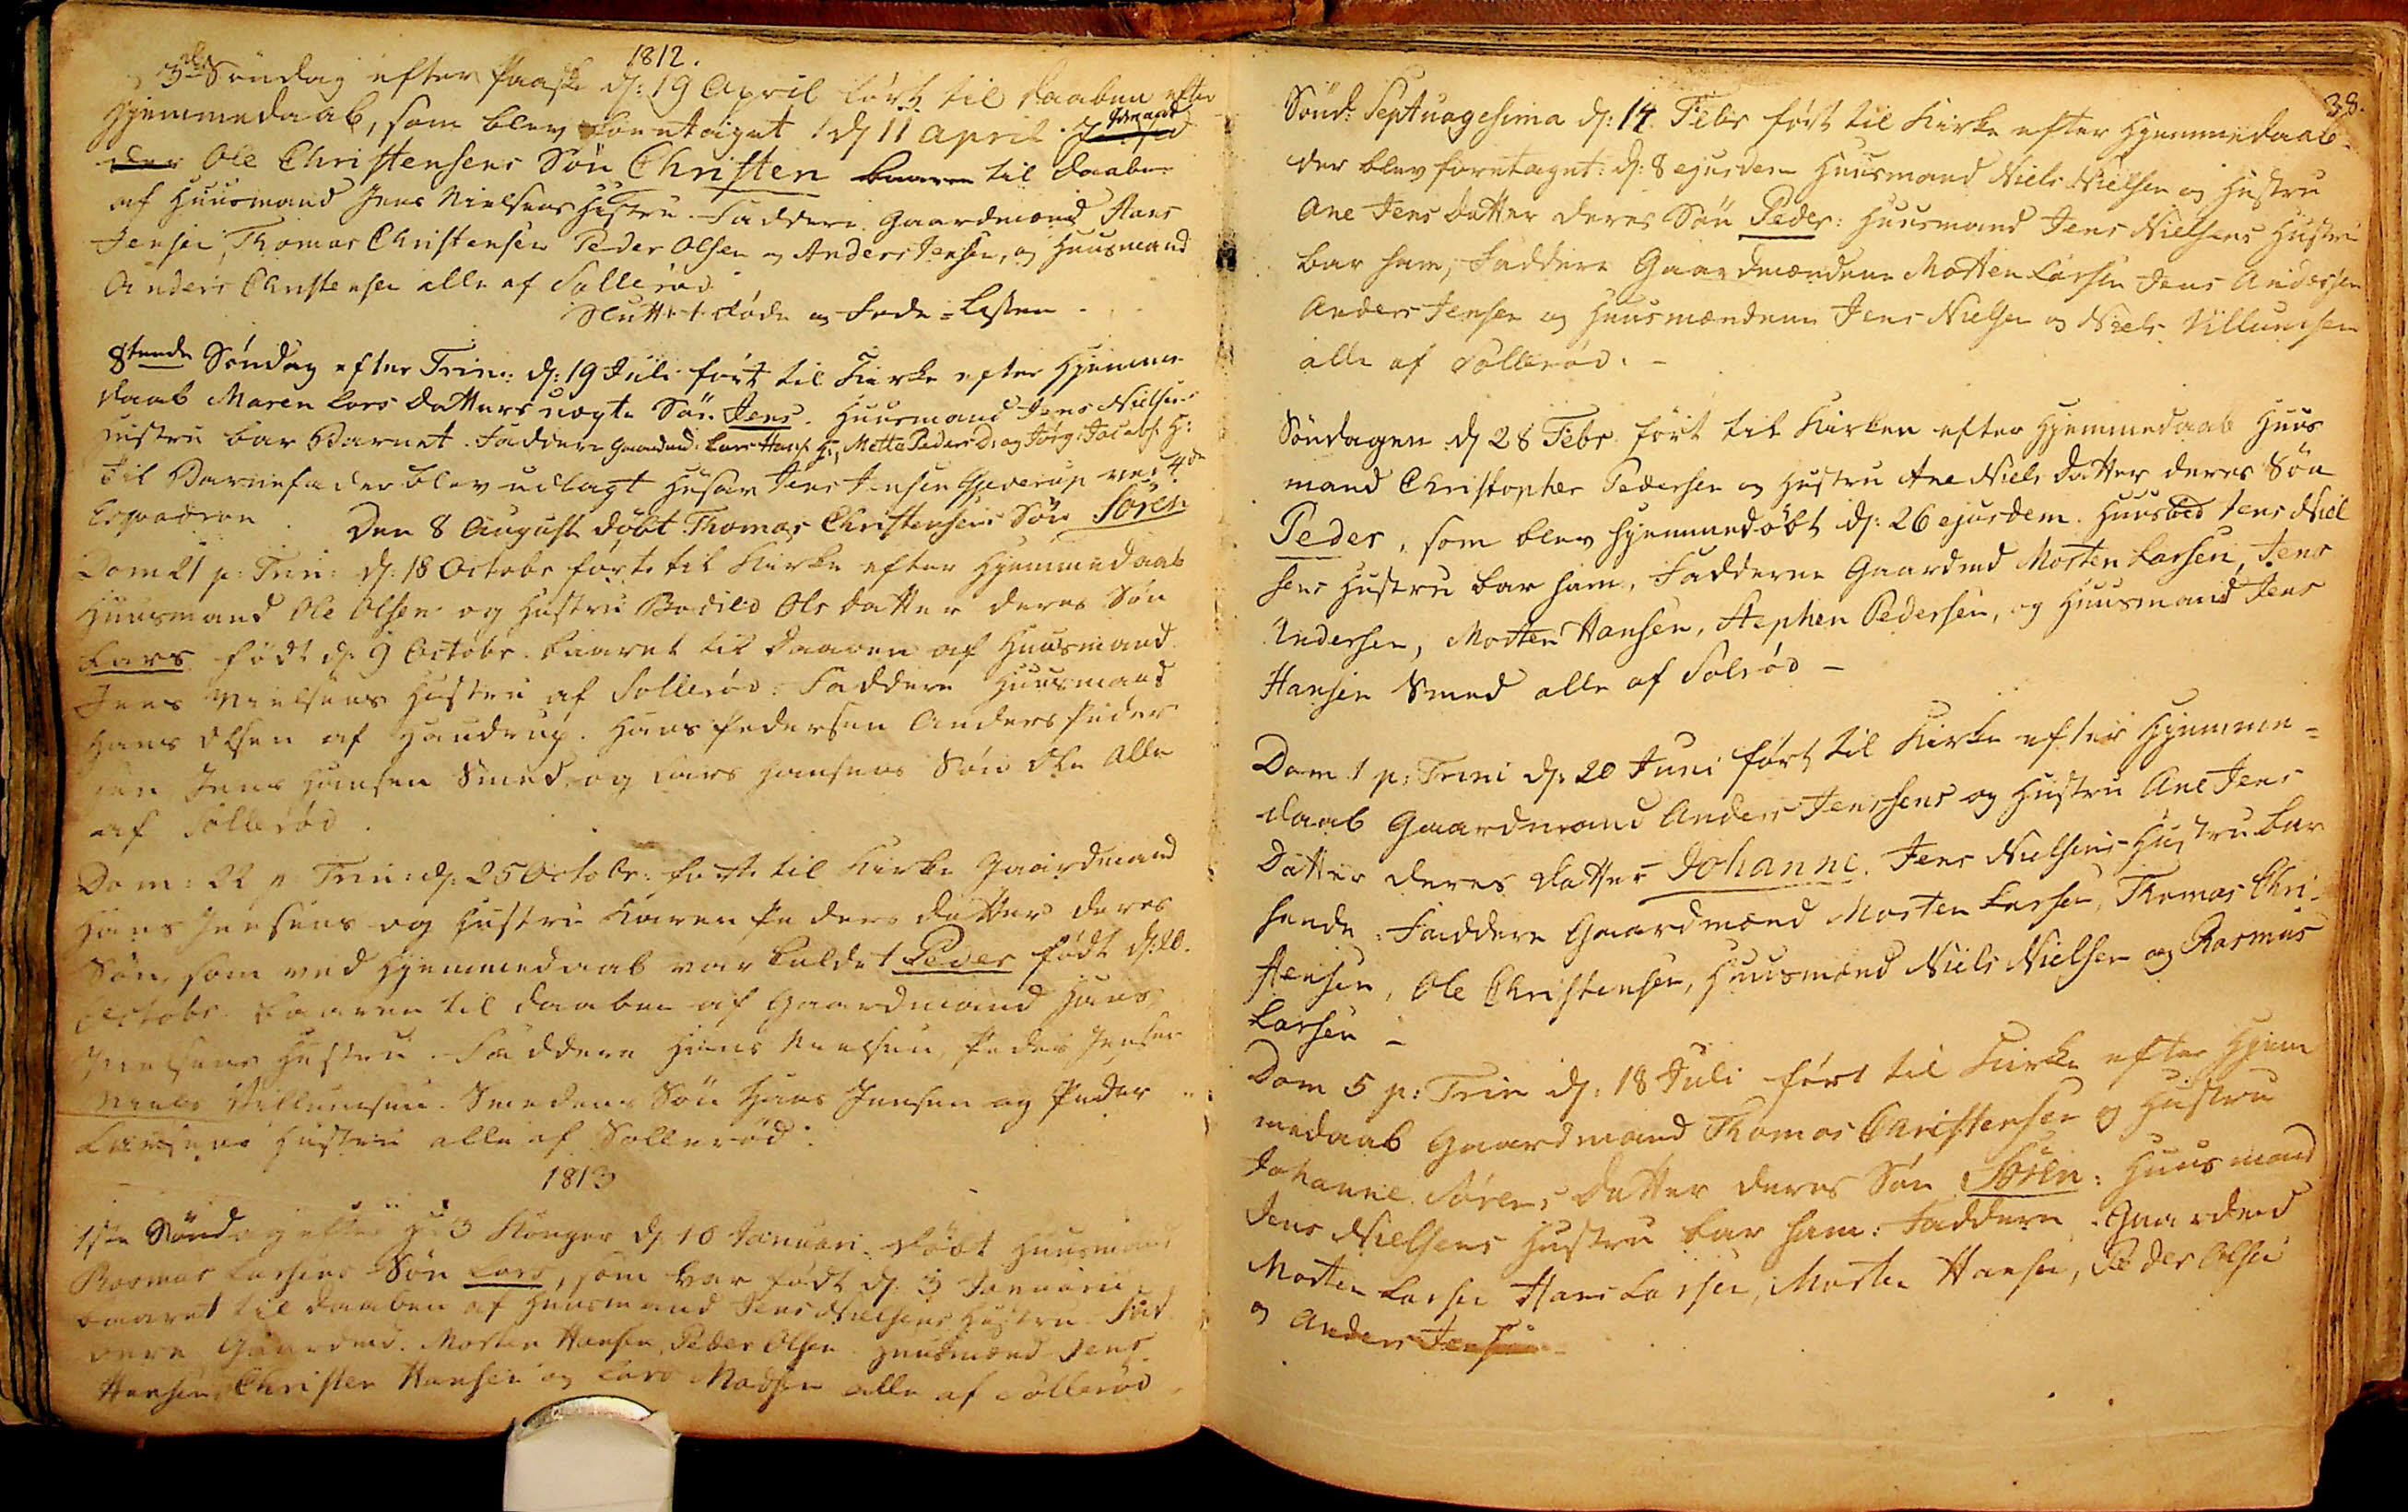1812.
3die Søndag efter Paaske d: 19 April ført til Daaben efter
Gdmand
Hjemmedaab, som blev foretaget d 11 April. Indsid
der Ole Christensens Søn Christen baaren til Daaben
af Huusmand Jens Nielsens Hustru. Faddere. Gaardmænd Hans
Jensen, Thomas Christensen Peder Olsen og Anders Jensen, og Huusmand
Anders Christensen alle af Sollerød.
Sluttet døde og føde i Listen.
8tende Søndag efter Trin: d: 19 Juli ført til Kirke efter Hjemme
daab Maren Lars Datters uægte Søn Jens. Huusmand Jens Nielsens
Hustru bar Barnet. Faddere Gaardmd: Lars Hans: H:, Mette Peders D: og Jørg: Jacobs: H:
Til Barnefader blev udlagt Husar Jens Jensen Gjederup ved 4de
Esqvadron. Den 8 August døbt Thomas Christensens Søn Søren
Dom 21 p: Trin: d: 18 Octobr førte til Kirke efter Hjemmedaab
Huusmand Ole Olsen og Hustru Bodild Ols Datter deres Søn
Lars. født d: 9 Octobr: baaret til Daaben af Huusmand
Jens Nielsens Hustru af Sollerød: Faddere Huusmand
Hans Olsen af Haudrup. Hans Pedersen Anders Peder
sen, Jens Hansen Smed og Lars Hansens Søn Ole Alle
af Sollerød
Dom: 22 p: Trin: d: 25 Octobr: førte til Kirke Gaardmand
Hans Jensens og Hustru Karen Peders Datter deres
Søn, som ved Hjemmedaab var kaldet Peder født d: 20.
Octobr baaren til daaben af Gaardmand Hans
Nielsens Hustru. Faddere Hans Nielsen, Peder Jensen
Niels Willumsen, Smedens Søn Hens Jensen og Peder
Larsens Hustru alle af Sollerød.
1813
1ste Søndag efter H: 3 Konger d: 10 Januari døbt Huusmand
Rasmus Larsens Søn Lars, som var født d: 3 Januari
baaret til Daaben af Huusmand Jens Nielsens Hustru. Fad
dere Gaardmd: Morten Hansen, Peder Olsen. Huusmænd Jens
Hansen, Christen Hansen og Lars Madsen alle af Sollerød.
38.
Sønd: Septuagesima d: 14. Febr ført til Kirke efter Hjemmedaab,
der blev foretaget: d: 8 ejusdem Huusmand Niels Nielsen og Hustru
Ane Jens Datter deres Søn Peder: Huusmand Jens Nielsens Hustru
bar ham; Faddere Gaardmændene Morten Larsen Jens Andersen
Anders Jensen og Huusmændene Jens Nielsen og Niels Villumsen
alle af Sollerød.
Søndagen d 28 Febr: ført til Kirken efter Hjemmedaab Huus
mand Christopher Pedersen og Hustru Ane Niels Datter deres Søn
Peder, som blev Hjemmedøbt d: 26 ejusdem. Huusmd Jens Niel
sens Hustru bar ham, Faddere Gaardmd Morten Larsen, Jens
Andersen, Morten Hansen, Stephen Pedersen, og Huusmand Jens
Hansen Smed alle af Solrød -
Dom 1 p: Trini d: 20 Juni ført til Kirke efter Hjemme¬
daab Gaardmand Anders Jensens og Hustru Ane Jens
Datter deres Datter Johanne. Jens Nielsens Hustru bar
hande. Faddere Gaardmænd Morten Larsen, Thomas Chri
stensen, Ole Christensen, Huusmænd Niels Nielsen og Rasmus
Larsen
Dom 5 p: Trin d: 18 Juli ført til Kirke efter Hjem
medaab Gaardmand Thomas Christensen og Hustru
Johanne Sørens Datter deres Søn Søren: Huusmand
Jens Nielsens Hustru bar ham: Faddere Gaardmd
Morten Larsen Hans Larsen, Morten Hansen, Peder Olsen
og Anders Jensen
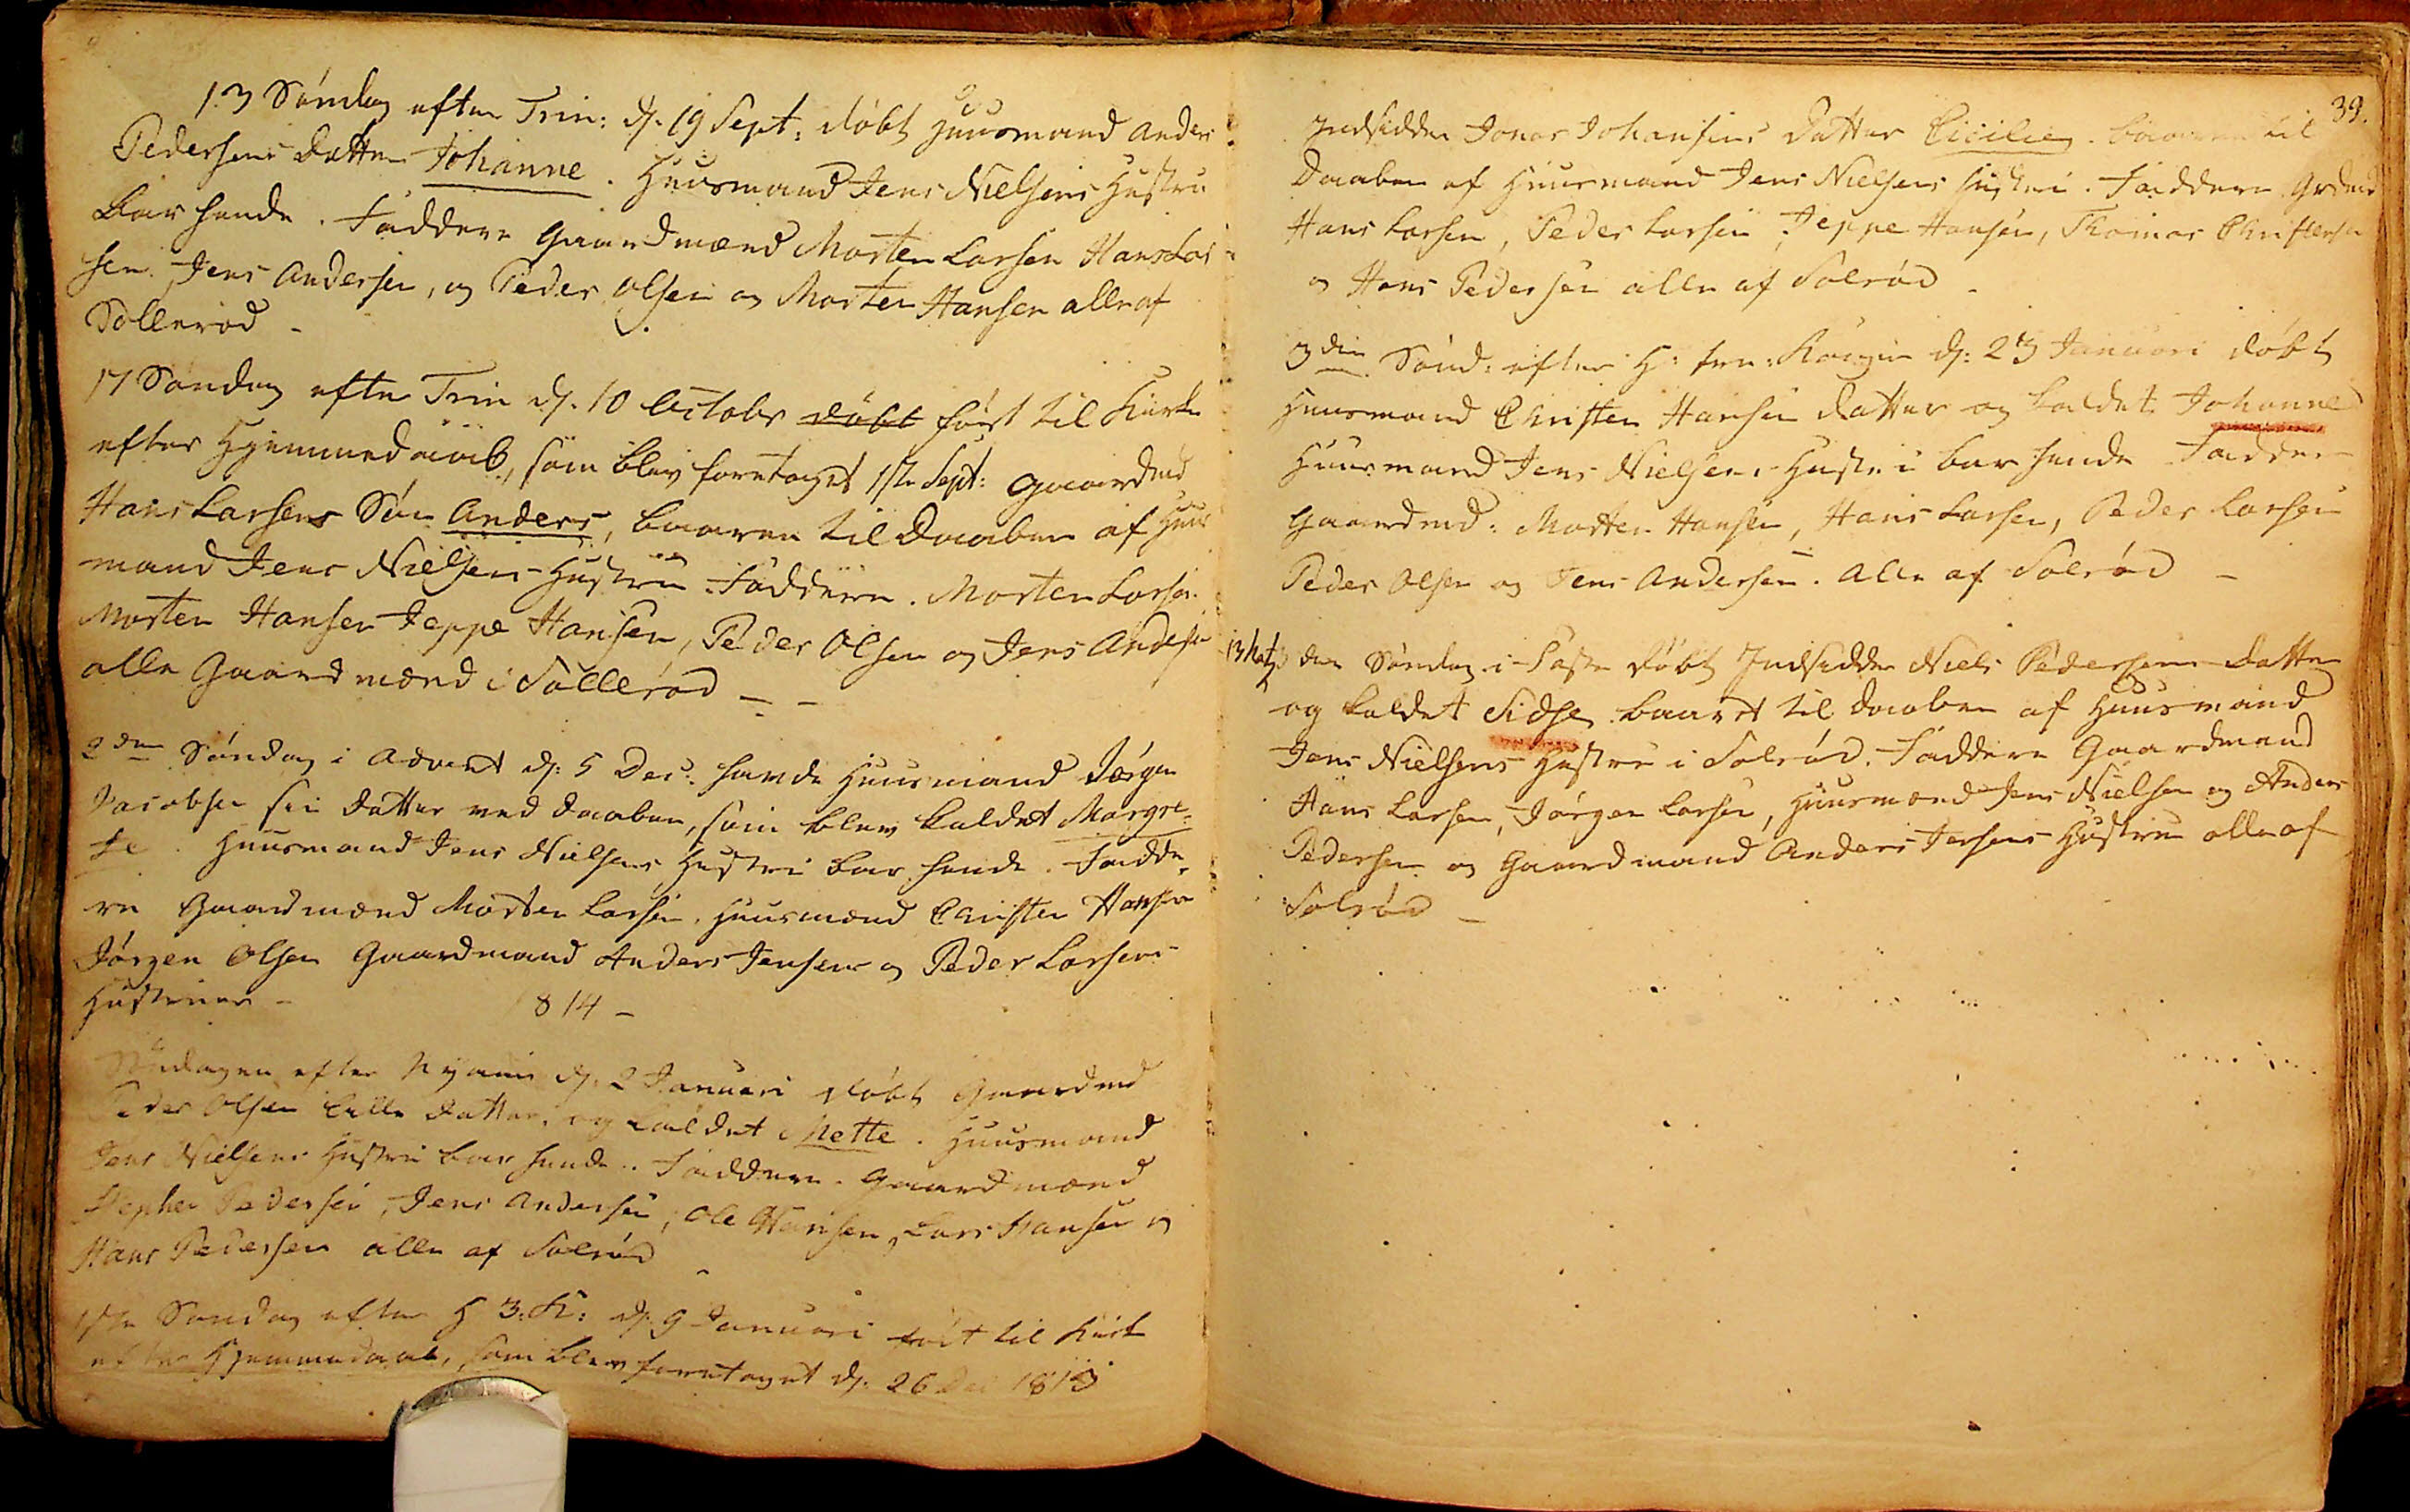13 Søndag efter trin: d: 19 Sept: døbt Huusmand Anders
Pedersens datter Johanne. Huusmand Jens Nielsens Hustru
bar hende. Faddere Gaardmænd Morten Larsen Hans Lar
sen. Jens Andersen, og Peder Olsen og Morten Hansen alle af
Sollerød
17 Søndag efter Trin d: 10 Octobr døbt ført til Kirke
efter Hjemmedaab, som blev foretaget 1ste Sept: Gaardmd
Hans Larsens Søn Anders, baaren til Daaben af Huus
mand Jens Nielsens Hustru. Faddere. Morten Larsen
Morten Hansen Jeppe Hansen, Peder Olsen og Jens Andersen
alle Gaardmænd i Sollerød
2den Søndag i Advent d: 5 Dec: havde Huusmand Jørgen
Jacobsen sin Datter ved Daaben, som blev kaldet Margre¬
te. Huusmand Jens Nielsens Hustru bar hende. Fadde¬
re Gaardmænd Morten Larsen, Huusmænd Christen Hansen
Jørgen Olsen Gaardmand Anders Jensens og Peder Larsens
Hustruer
1814
Søndagen efter Nyaar d: 2 Januari døbt Gaardmd
Peder Olsen lille Datter, og kaldet Mette. Huusmand
Jens Nielsens Hustru bar hende. Faddere. Gaardmænd
Stephen Pedersen, Jens Andersen, Ole Hansen, Lars Hansen og
Hans Pedersen alle af Solrød -
1ste Søndag efter H 3: K: d: 9 Januari ført til Kirke
efter Hjemmedaab, som blev foretaget d: 26 Dec: 1813
39.
Indsidder Jonas Johansens Datter Cicilie. baaren til
Daaben af Huusmand Jens Nielsens Hustru. Faddere. Grdmd
Hans Larsen, Peder Larsen, Jeppe Hansen, Thomas Christensen
og Hans Pedersen alle af Solrød
3die Sønd: efter H: tre Konger d: 23 Januari døbt
Huusmand Christen Hansen Datter og kaldet Johanne
Huusmand Jens Nielsens Hustru bar hende. Faddere
Gaardmd: Morten Hansen, Hans Larsen, Peder Larsen
Peder Olsen og Jens Andersen. Alle af Solrød
13 Marts 3die Søndag i Faste døbt Indsidder Niels Pedersens Datter
og kaldet Sidse. baaret til Daaben af Huusmand
Jens Nielsens Hustru i Solrød. Faddere Gaardmand
Hans Larsen, Jørgen Larsen, Huusmænd Jens Nielsen og Anders
Pedersen og Gaardmand Anders Jensens Hustru alle af
Solrød